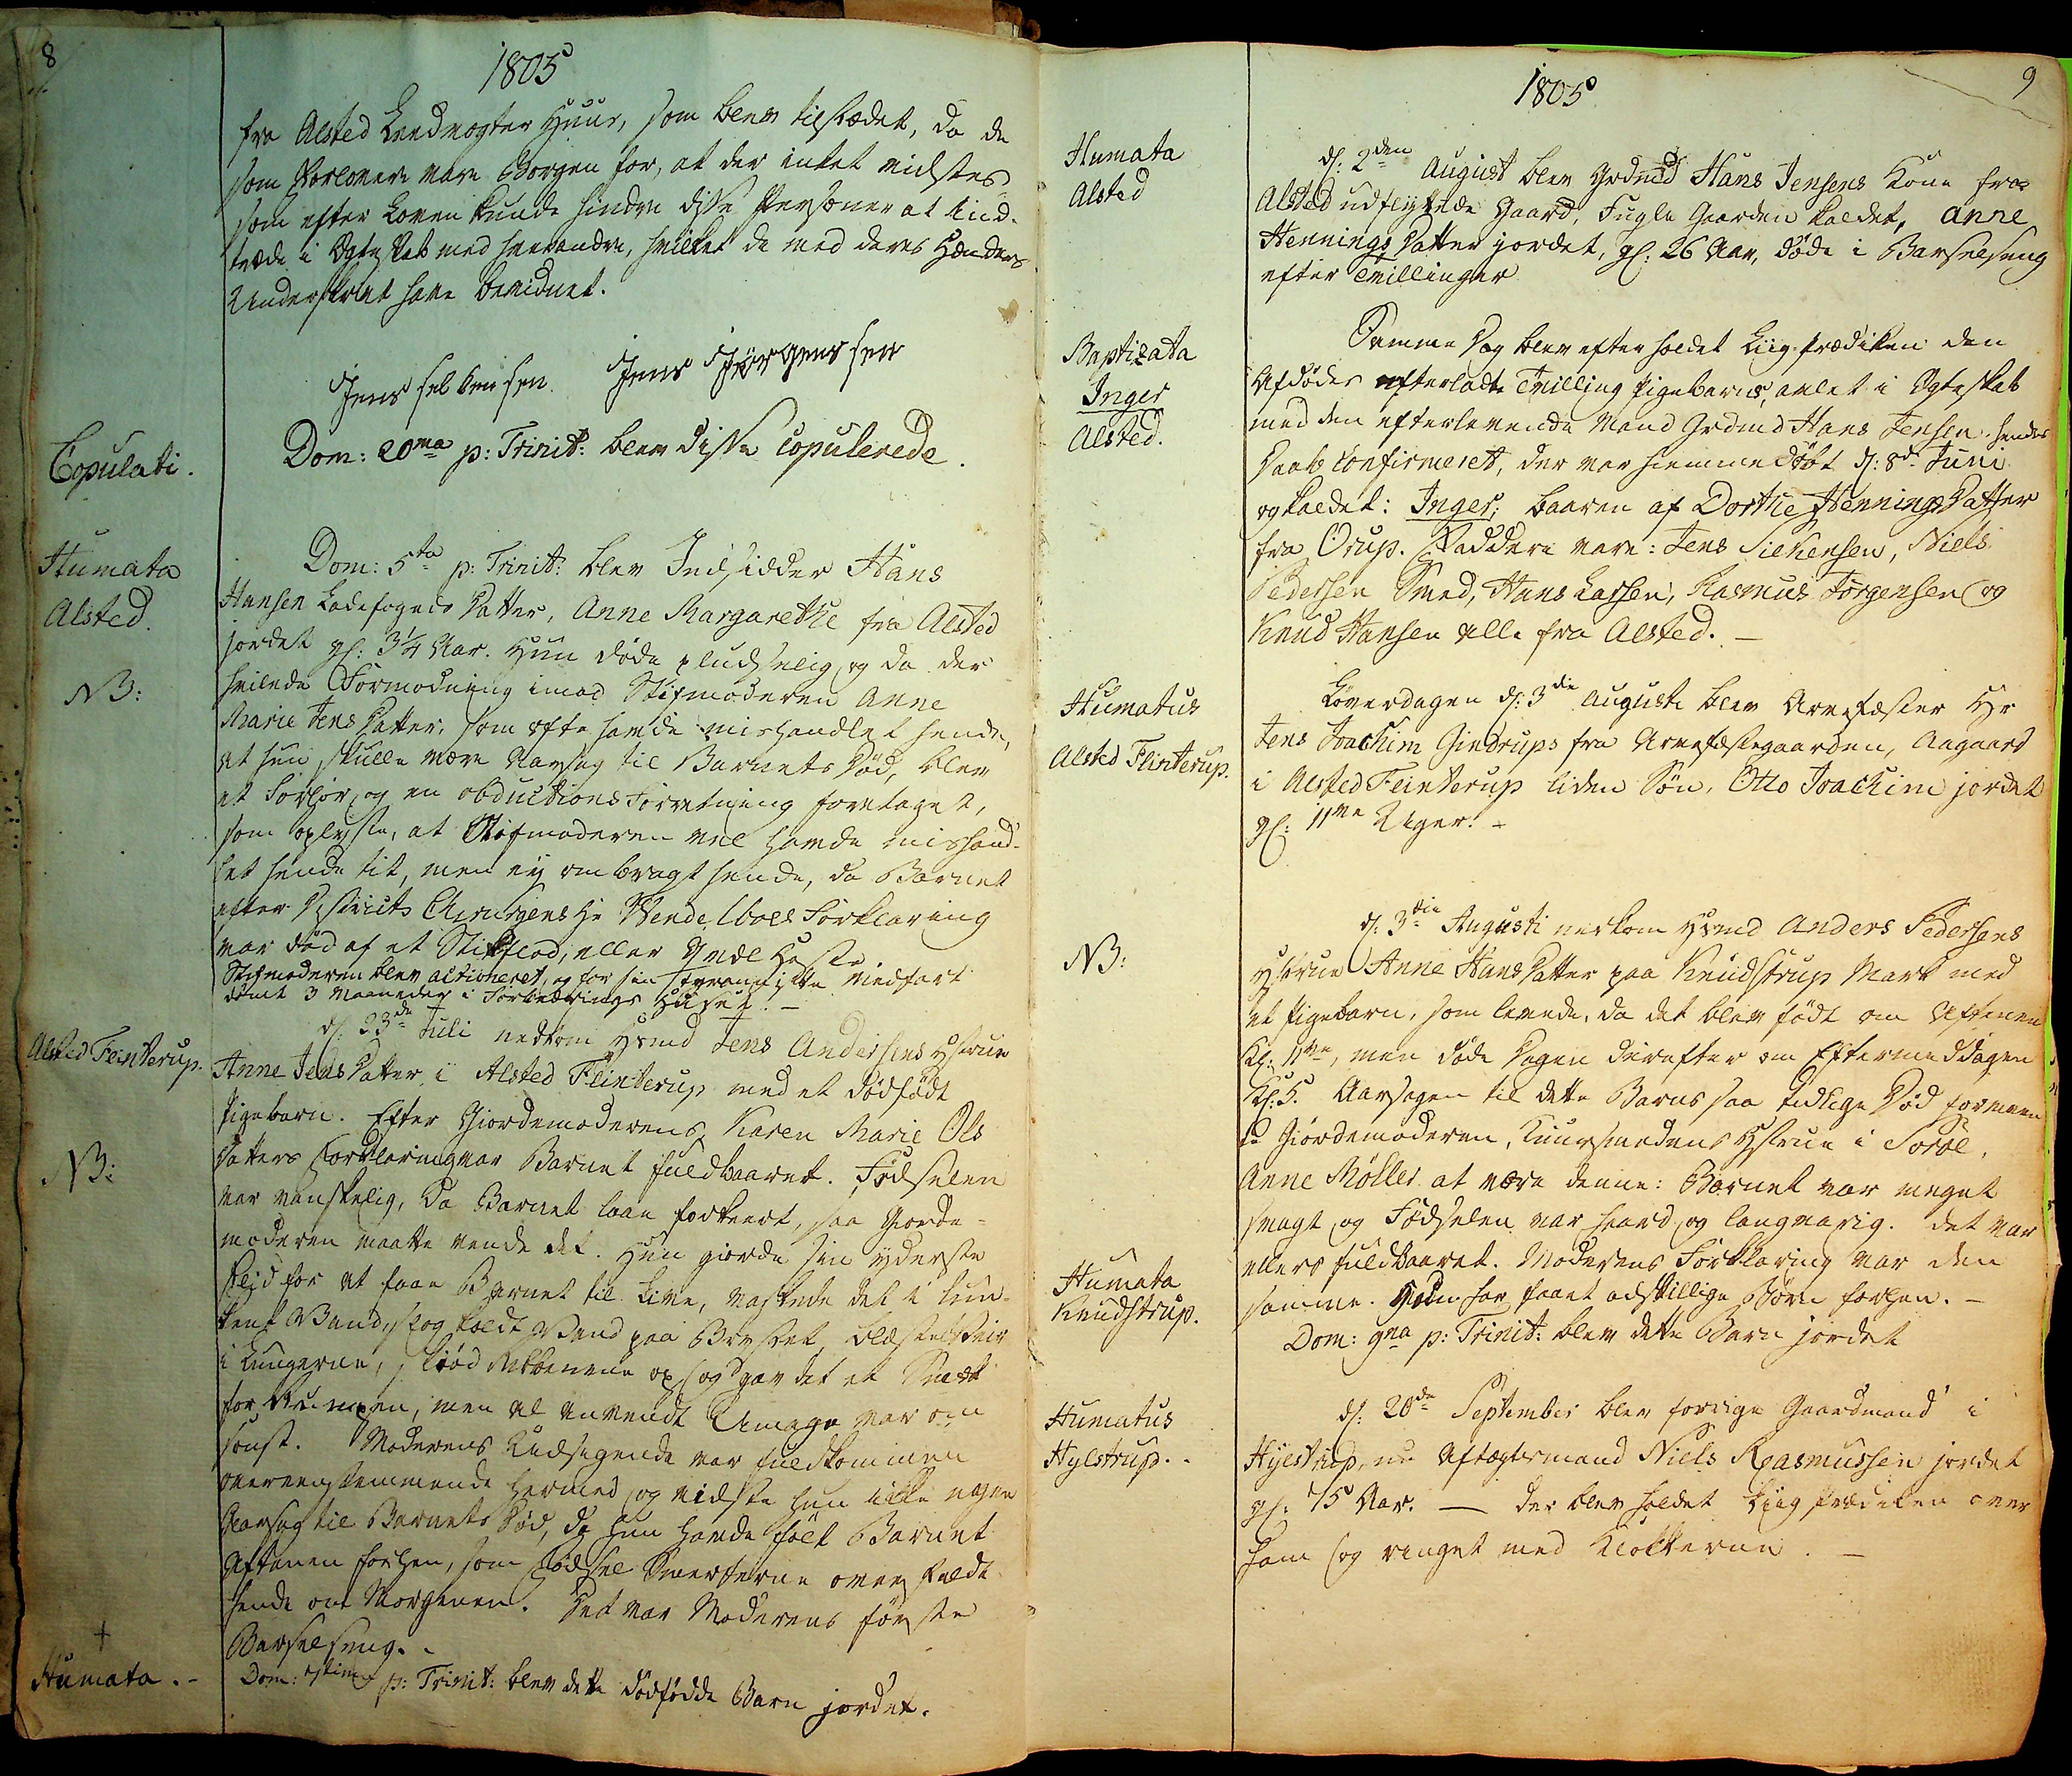18.
Copulati.
Humata
Alsted.
NB:
Alsted Flinterup.
NB:
Humata.
1805
fra Alsted Leedvogter Huus, som blev tilstædet, da de
som forlovere vare Borgen for, at der intet vidstes
som efter Loven kunde hindre disse Personer at ind¬
træde i Ægteskab med hverandre, hvilket de med deres Hænders
Underskrivt have bevidnet.
Jens selkensen. Jens Jørgenssens
Dom: 20ma p: Trinit: blev disse copulerede.
Dom: 5ta p: Trinit: blev Indsidder Hans
Hansen Ladefogeds Datter, Anne Margarethe fra Alsted
jordet gl: 3¼ Aar. Hun døde pludselig, og da der
hvilede Formodning imod Stifmoderen Anne
Marie Jens Datter, som oftye havde mihandlet hende,
at hun skulle være Aarsag til Barnets Død, blev
et Forl´hør og en obductions Forretning foretaget,
som oplyste, at Skoemoderen vel havde mishand¬
let hende tit, men ey ombragt hende, da Barnet
efter Distriits Chirurgens Hr Wendelboes Forklaring
var død af et Stikflod, eller Qvdl Hoste.
Stifmoderen blev actioneret, og for sin tyranni## ##e ##edfodt
dømt 3 Maaneder i Forbedrings Huset
d. 23de Juli nedkom Hsmd Jens Andersens Hstrue
Anne Jens Datter i Alsted Flinterup med et dødfødt
Pigebarn. Efter Giordemoderens Karen Marie Ols
Datters Forklaring var Barnet fuldbaaret. Fødselen
var vaskelig, da Barnet laa forkeedt, saa Giorde¬
moderen maatte vende det. Hun giorde sin yderste
Flid for at faae Barnet til Live, vaskede det i lun¬
kent Vand, og koldt Vand paa Brystet, blæste ##stei##
i Lungerne, skiød Ribbenene op og gav det et Sm##
for Rumpen, men al anvendt Umage, var om¬
sonst. Moderens Udsegende var fuldkommen
overensstemmende hermed og vidste hun ikke nogen
Aarseg til Barnets Død, da hun havde følt Barnet
Aftenen forhen, som Fødsel Smerterne overfaldt
hende om Morgenen. Det var Moderens første
Barselseng.
Dom: 7tima p: Trinit: blev dette dødfødde Barn jordet.
Humata
Alsted
Baptizata
Inger
Alsted.
Humatus
Alsted Flinterup.
NB:
Humata
Knudstrup
Humatus
Hylstrup.
9.
1805
d: 2den August blev Grdmd Hans Jensens Kone fra
Alsted udflyttede Gaard, Fugle Gaarden kaldet, Anne
Hennings Datter jordet gl: 26 Aar, døde i Barselseng
efter Tvillinger.
Samme Dag blev efter holdet Liig=prædiken den
Afdødes efterladte Tvilling Pigebarns, avlet i Ægteskab
med den efterlevende Mand Grdmd Hans Jensen, hendes
Daab Confirmeret, der var hiemmedøbt d: 8de Juni
og kaldet: Inger; baaren af Dorthe Hennings Datter
fra Orup. Faddere vare: Jens Silkensen, Niels
Pedersen Smed, Hans Lassen, Rasmus Jørgensen og
Knud Hansen alle fra Alsted.
Løverdagen d: 3die Augusti blev Arvefæster Hr
Jens Joachim Gindrups fra Arvefæstegaarden, Aagaard
i Alsted Flinterup liden Søn, Otto Joachim jordet
gl: 11ma Uger.
d: 3die Augusti nedkom Hsmd Anders Pedersens
Hstrue Anne Hans Datter paa Knudstrup Mark med
et Pigebarn, som levede, da det blev født om Aftenen
kl: 11me, men døde Dagen derefter om Eftermiddagen
Kl: 5 Aarsagen til dette Barns saa tidlige Død for##
te Giordemoderen Kiur##dens Hstrue i Sorøe
Anne Møller at være denne; Barnet var meget
svagt og Fødselen var haard og langvarig, det var
ellers Fuldbaaret. Moderens Forklaring var den
samme. Hun har faaet adskillige Børn, forhen.
Dom: 9na p: Trinit: blev dette Barn jordet
d. 20de September blev forrige Gaardmand i
Hylstrup, nu Aftægtsmand Niels Rasmussen jordet
gl: 75 Aar. - der blev holdet Liigprædiken over
ham og ringet med Klockerne
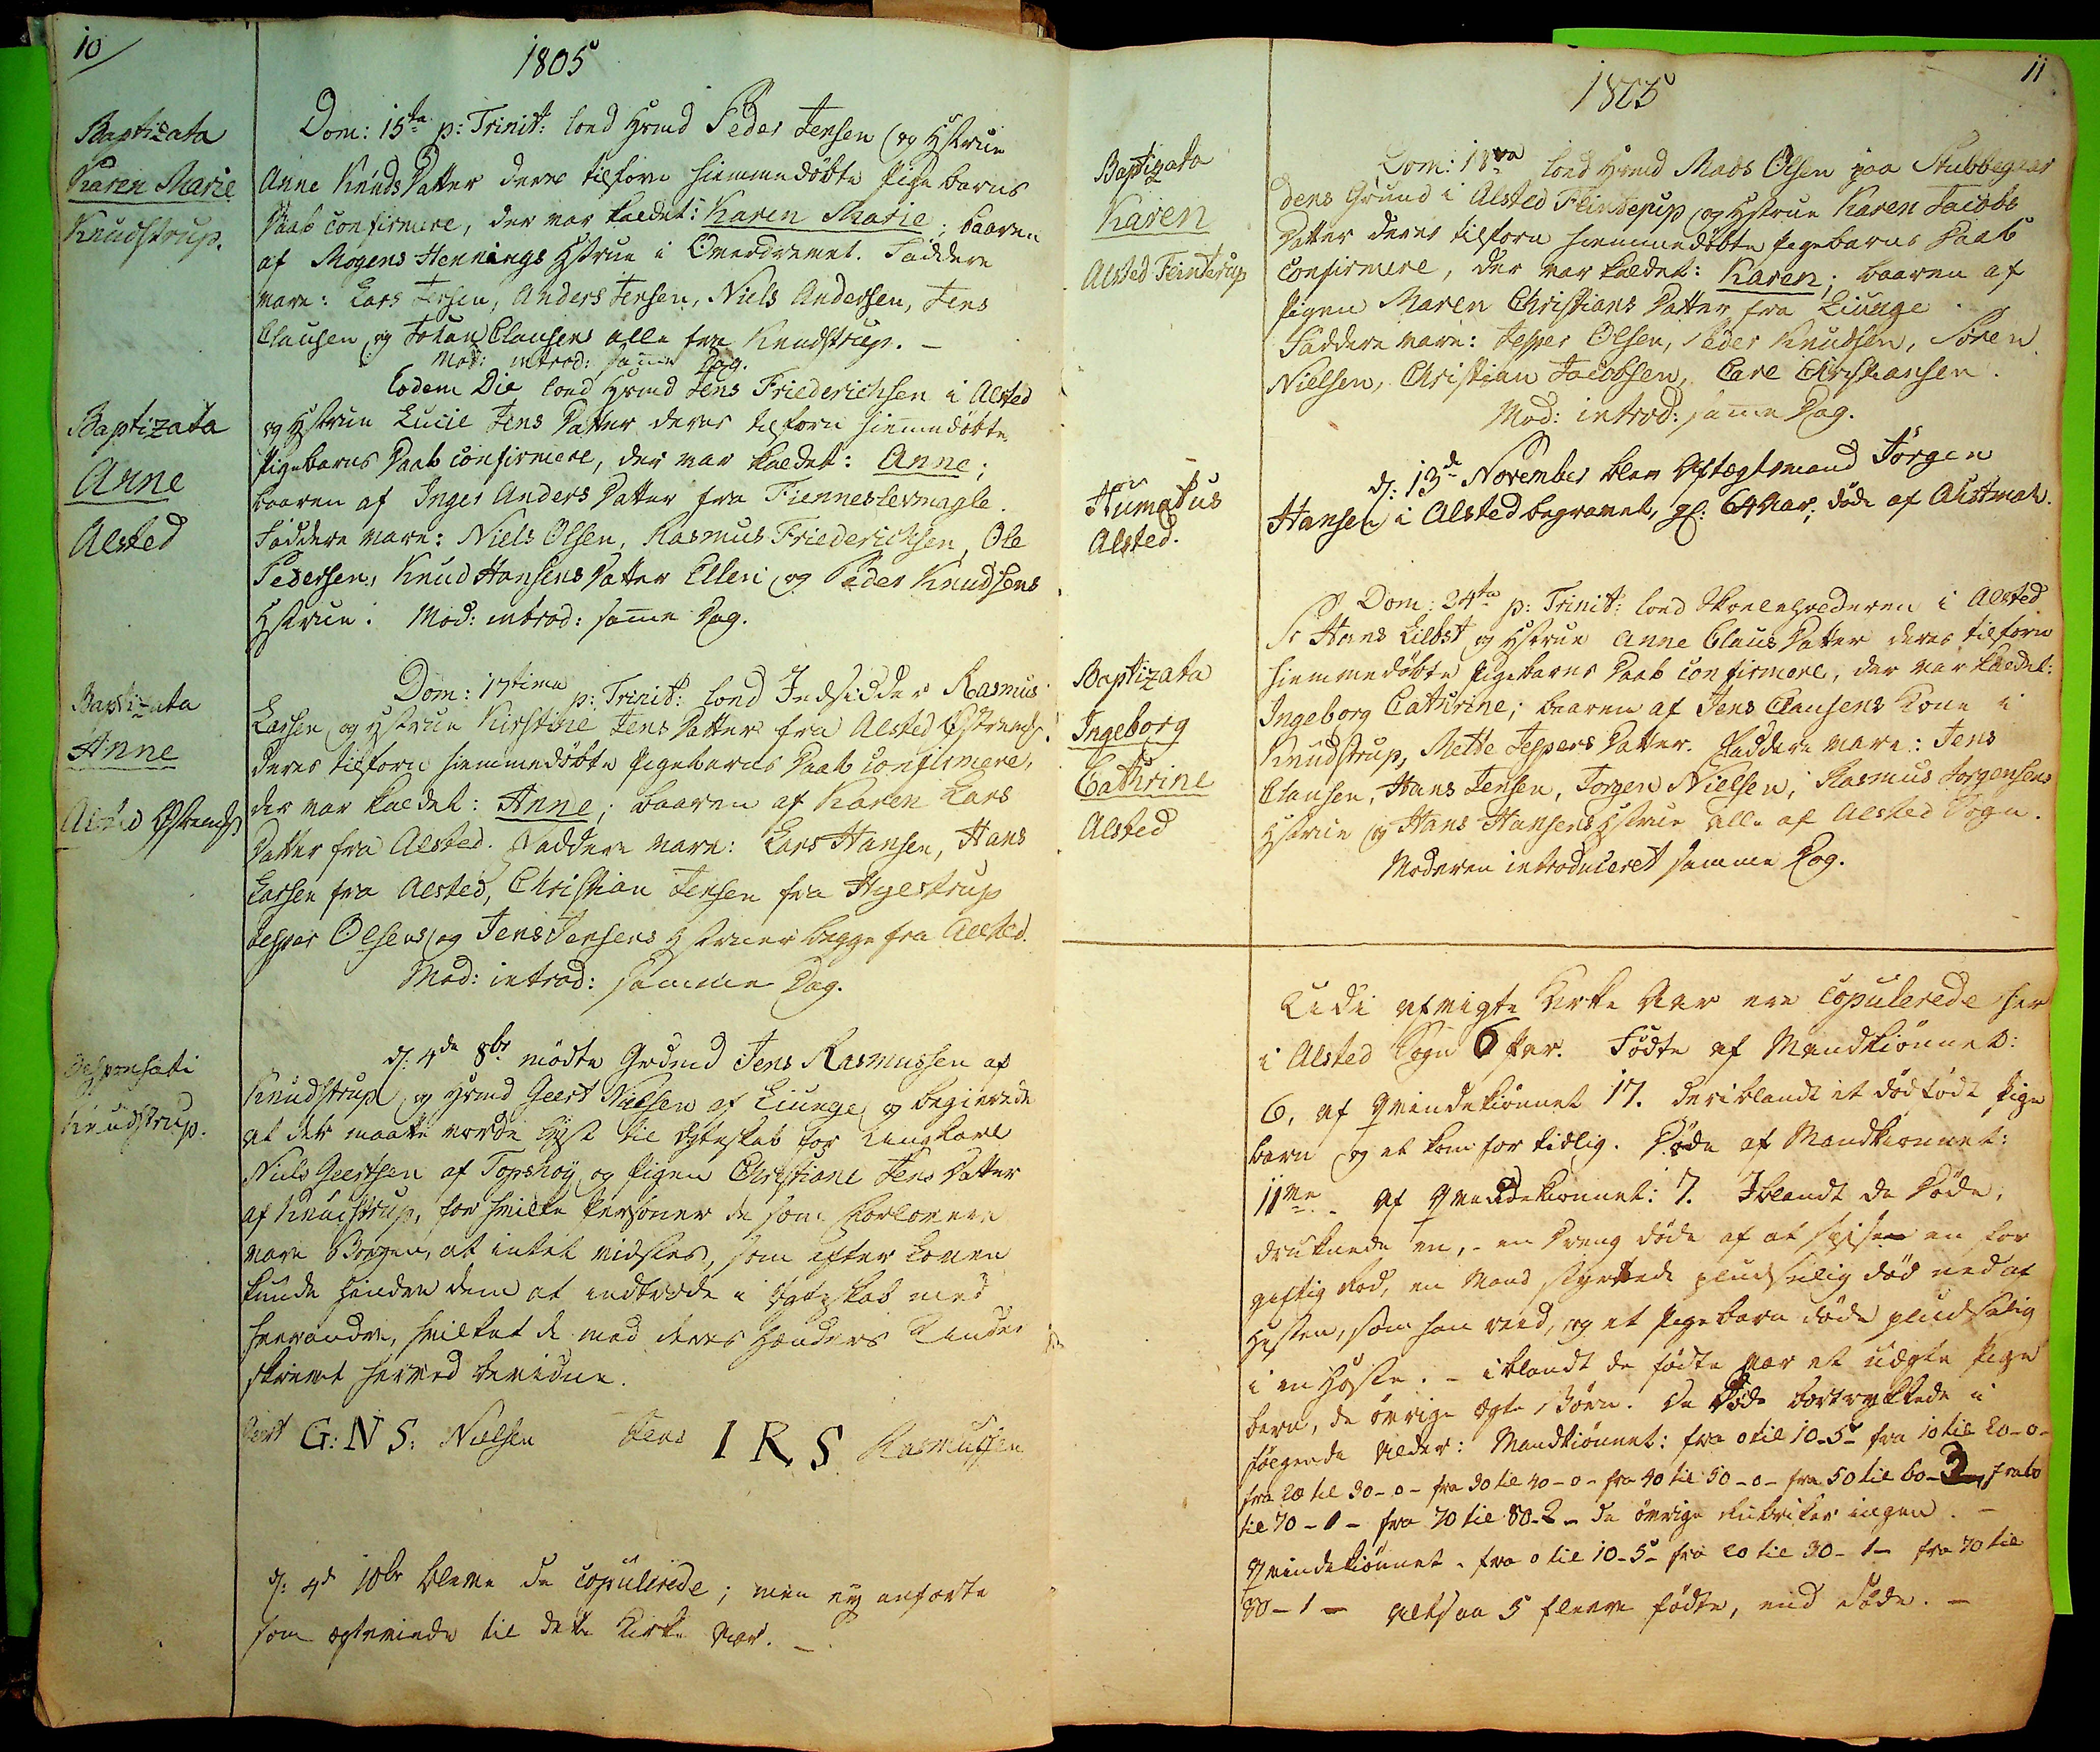10.
Baptizata
Karen Marie
Knudstrup.
Baptizata
Anne
Alsted
Baptizata
Anne
Alsted Østrem##
Desponsati
Knudstrup.
1805
Dom: 15ta p: Trinit: loed Hsmd Peder Jensen og Hstrue
Anne Knuds Datter deres tilforn hiemmedøbte Pige barns
Daab confirmere, der var kaldet: Karen Marie; baaren
af Mogens Hennings Hstrue i Overdrevet. Faddere
vare: Lars Jensen, Anders Jensen, Niels Andersen, Jens
Clausen, og Johan Clausen alle fra Knudstrup.
Mod: introd: samme Dag.
Eodem Die loed Hsmd Jens Friderichsen i Alsted
og Hstrue Lucie Jens Datter deris tilforn hiemmedøbte
Pigebarns Daab confirmere, der var kaldet: Anne;
baaren af Inger Anders Datter fra Fienneslevmagle.
Faddere vare: Niels Olsen, Rasmus Friderichsen, Ole
Pedersen, Knud Hansens Datter Ellen og Peder Knudsens
Hstrue. Mod: introd: samme Dag.
Dom: 17tima p: Trinit: loed Indsidder Rasmus
Larsen, og Hstrue Kirstine Jens Datter fra Alsted Østrem##
deres tilforn hiemmedøbte Pigebarns Daab confirmere,
der var kaldet: Anne; baaren af Karen Lars
Datter fra Alsted. Faddere vare: Lars Hansen, Hans
Larsen fra Alsted, Christian Jensen fra Hylstrup
Jesper Olsens og Jens Jensens Hstruer begge fra Alsted.
Mod: introd: samme Dag.
d: 4de 8br mødte Grdmd Jens Rasmussen af
Knudstrup, og Hsmd Geert Nielsen af Liunge og begierede
at der maatte vorde lyst til Ægteskab for Ungkarl
Niels Geertsen af Topshøy, og Pigen Christiane Jens Datter
af Knudstrup, for hvilke Personer de som Forlovere
vare Borgen, at intet vidstes, som efter Loven
kunde hindre dem at indtræde i Ægteskab med
hverandre, hvilket de med deres Hænders Linder
skrivt herved bevidne
Geert G: N S: Nielsen Jens I R S Rasmussen
d. 4de 10br bleve de copulerede; men ny anforte
som ægteviede til dette Kirke Aar.
Baptizata
Karen
Alsted Flinterup
Humatus
Alsted.
Baptizata
Ingeborg
Cathrine
Alsted
11.
1805
Dom: 18## loed Hsmd Mads Olsen paa Stubbegaar
dens Grund i Alsted Flinterup, og Hstrue Karen Jacobs
Datter deres tilforn hiemmedøbte Pigebarns Daab
confirmere, der var kaldet: Karen; baaren af
Pigen Maren Christians Datter fra Liunge
Faddere vare: Jesper Olsen, Peder Knudsen, Søren
Nielsen, Christian Jacobsen, Carl Christiansen
Mod: introd: samme Dag.
d: 13de November blev Aftægtsmand Jørgen
Hansen i Alsted begravet, gl: 64 Aar. døde af Astmah.
Dom: 24ta p: Trinit: loed Skoeleholderen i Alsted
S. Hans Liebst og Hustrue Anne Claus Datter deres tilforn
hiemmedøbte Pigebarns Daab confirmere, der var kaldet:
Ingeborg Cathrine; baaren af Jens Clausens Kone i
Knudstrup, Mette Jespers Datter. Faddere vare: Jens
Clausen, Hans Jensen, Jørgen Nielssen, Rasmus Jorgensens
Hstrue og Hans Hansens Hustrue, alle af Alsted Sogn.
Moderen introduceret samme Dag.
Udi afvigte Kirke Aar ere copulerede, her
i Alsted Sogn 6 Par. Fødte af Mandkiønnret:
6, af Qvindekiønnet 17. Derilandt et dødfødt Pige
barn og et kom for tidlig. Døde af Mandkionnet:
11me af Quindekionnet: 7. Iblandt de døde
druknede en, en Dreng døde af at spise en for
giftig Rod, en Mand styrterde pludselig død ned af
Hesten, som han red, og et Pigebarn døde pludselig
i en Hoste. Iblandt de fødte var et uægte Pige
barn, de berige ægte Børn. De Døde bortrykkede i
følgende Alder: Mandkionnet: fra 0 til 10 - 5 - fra 10 til 20 - 0 -
fra 20 til 30 - 0 - fra 30 til 40 - 0 - fra 40 til 50 - 0 - fra 50 til 60 - 3 - fra 60
til 70 - 1 - fra 70 til 80 - 2 de øvrige Rubriker ingen
Qvindekiønnet fra 0 til 10 - 5 - fra 20 til 30 - 1 - fra 70 til
80 - 1- Altsaa 5 flere fødte, end døde.